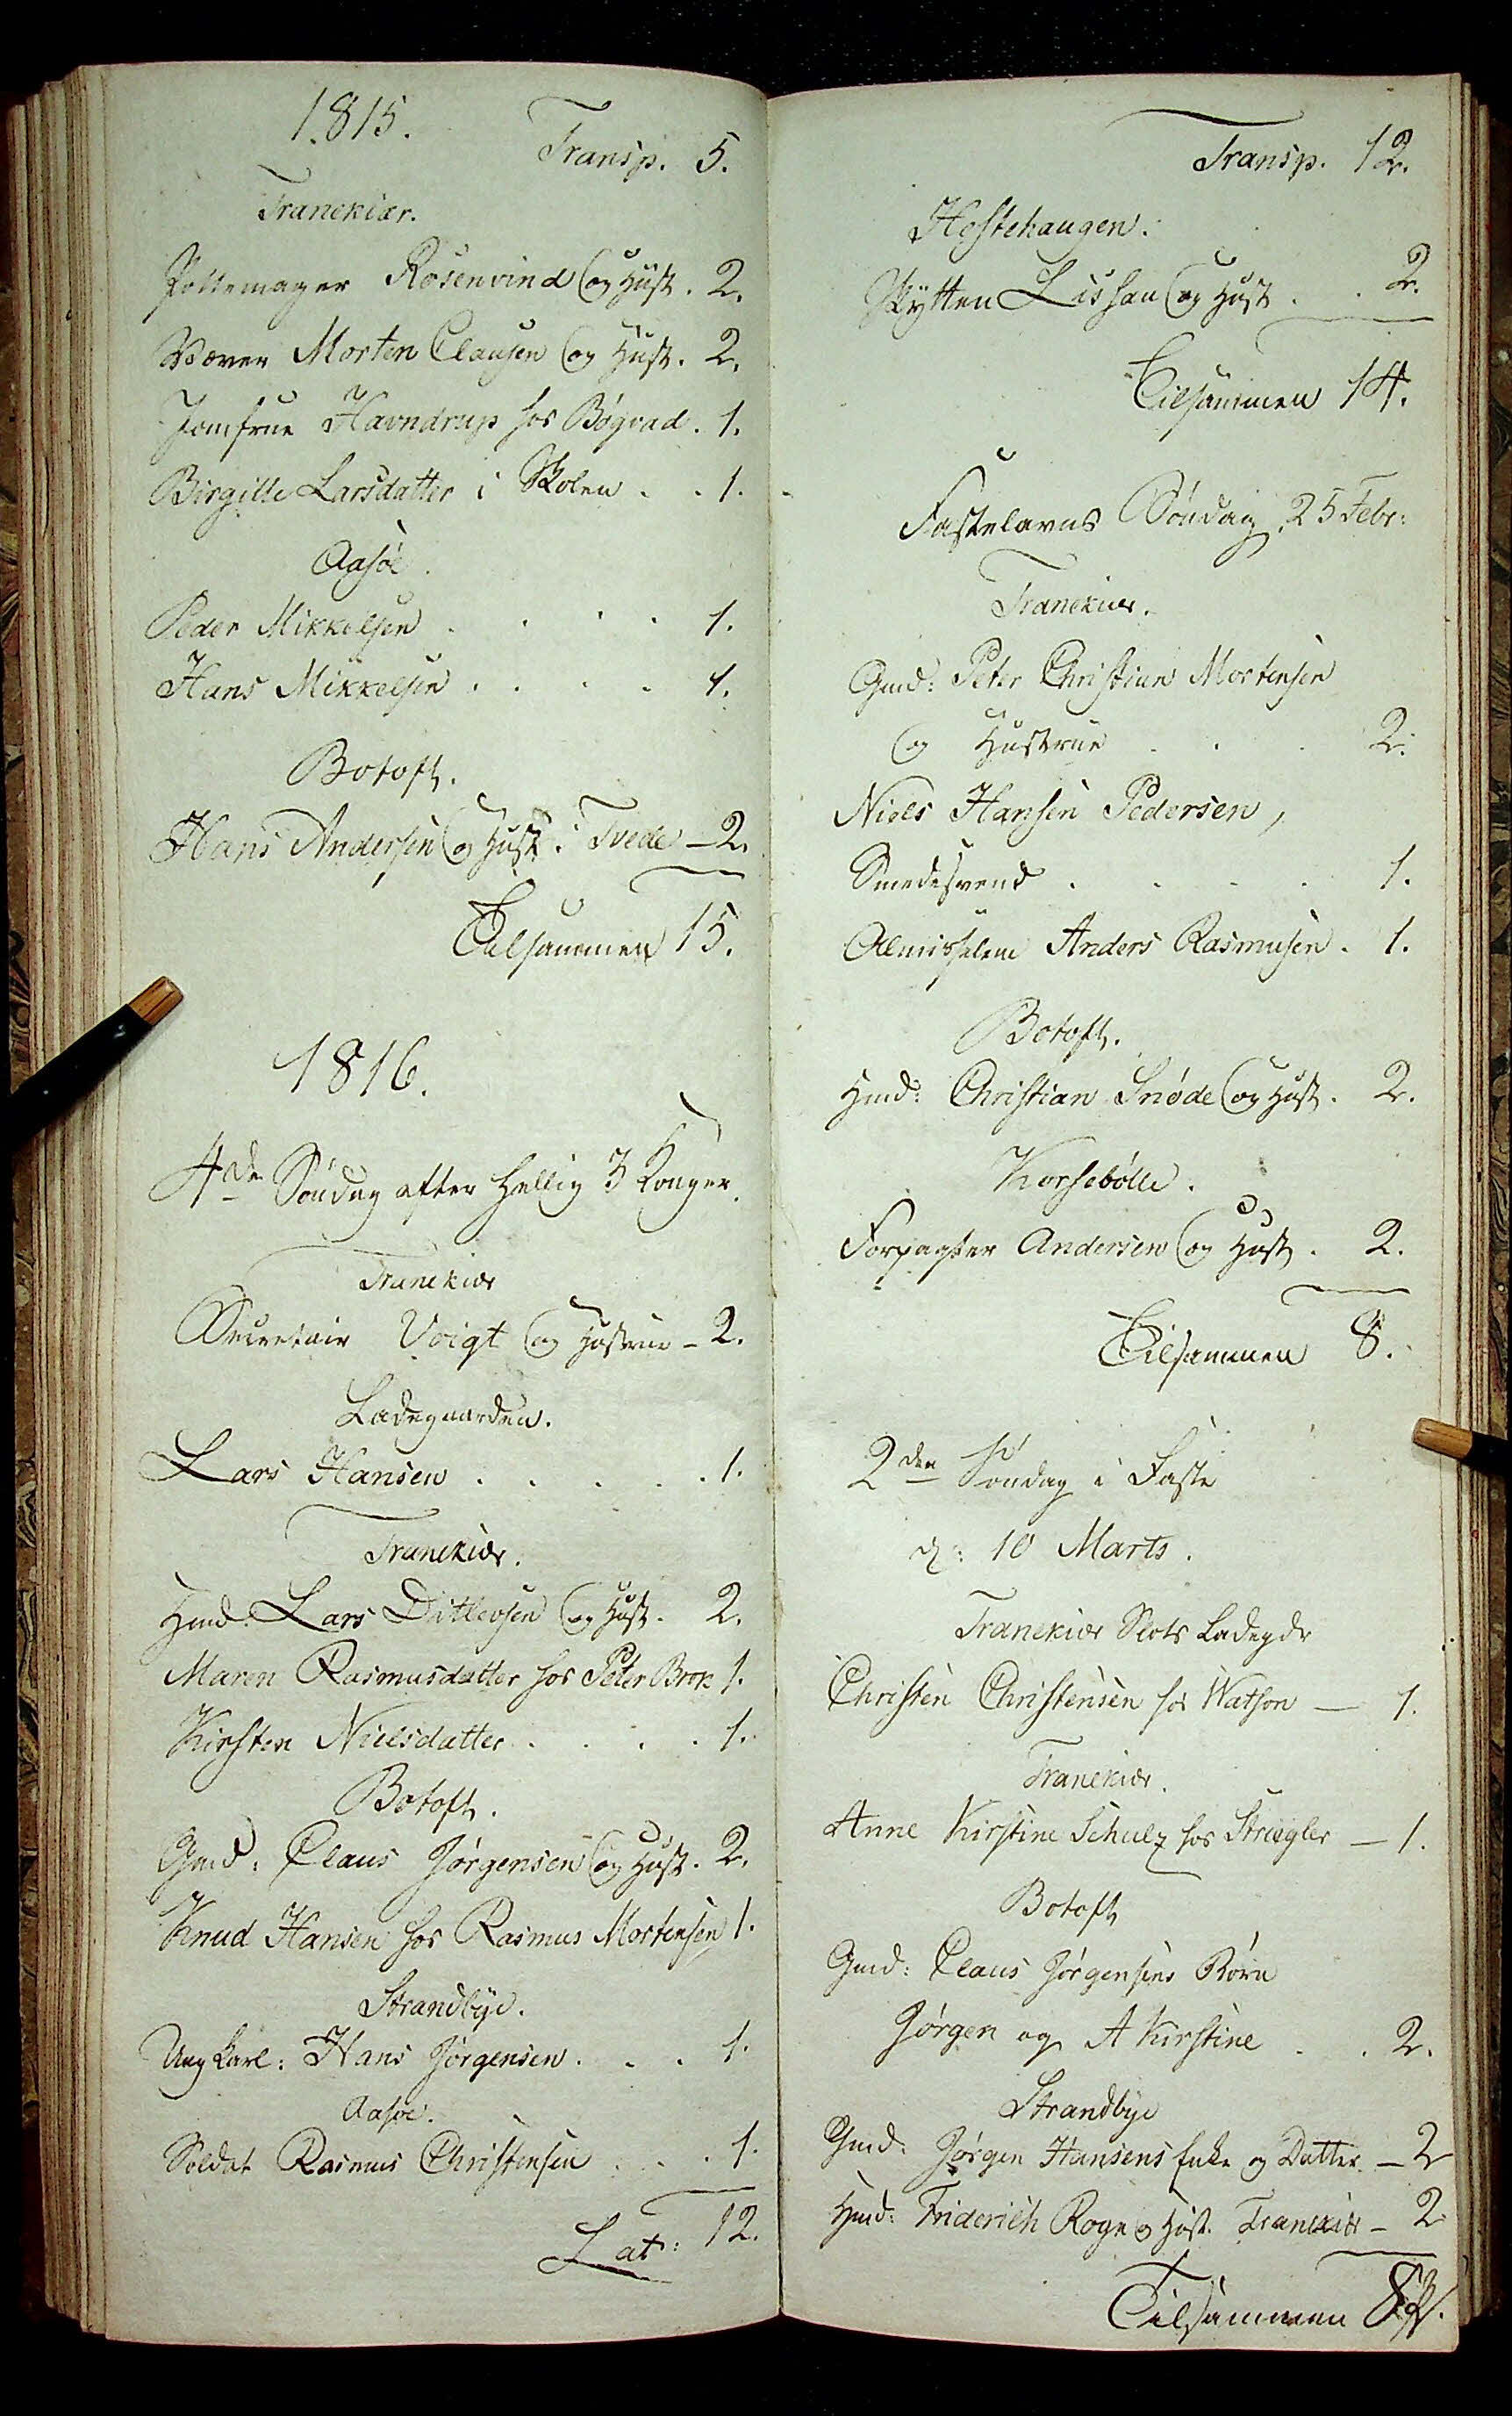1815.
Transp. 5.
Tranekiær.
Pottemager Rosenvind og Hust . 2.
Væver Morten Clausen og Hust . 2.
Jomfrue Havndrup hos Bøgvad . 1.
Birgitte Larsdatter i Skolen . . 1.
Aasøe
Peder Mikkelsen . . . . 1.
Hans Mikkelsen . . . . 1.
Botoft.
Hans Andersen og Hustr i Tvede - 2.
Tilsammen 15.
1816.
4de Søndag efter Hellig 3 Konger
Tranekiær
Secretair Voigt og Hustrue - 2.
Ladegaarden.
Lars Hansen . . . . . 1.
Tranekiær.
Hmd. Lars Ditlevsen og Hust . 2.
Maren Rasmusdatter hos Peter Brok 1.
Kirsten Nielsdatter . . . . 1.
Botoft.
Gmd: Claus Jørgensen og Hust . 2.
Knud Hansen hos Rasmus Mortensen 1.
Strandbye.
Ungkarl: Hans Jørgensen . . . 1.
Aasoe.
Soldat Rasmus Christensen . . . 1
Lat: 12
Transp. 12.
Hestehaugen.
Skytten Lissau og Hust . . 2.
Tilsammen 14.
Fastelavns Søndag 25 Febr:
Tranekiær.
Gmd: Peter Christian Mortensen
og Hustrue . . . 2.
Niels Hansen Pedersen,
Smedesvend . . . . 1.
Almisselem Anders Rasmusen . 1.
Botoft.
Hmd: Christian Snøde og Hust . 2.
Korsebølle.
Forpagter Andersen og Hust . 2.
Tilsammen 8.
2den Søndag i Faste
d: 10 Marts.
Tranekiær Slots Ladegdr
Christen Christensen hos Watson - 1.
Tranekiær
Anne Kirstine Schulz hos Striegler - 1.
Botoft
Gmd: Claus Jørgensens Børn
Jørgen og A Kirstine . . 2.
Strandbye
Gmd: Jørgen Hansens Enke og Datter - 2
Hmd: Friderich Rogn og Hust Tranekiær - 2.
Tilsammen 8 Ps.
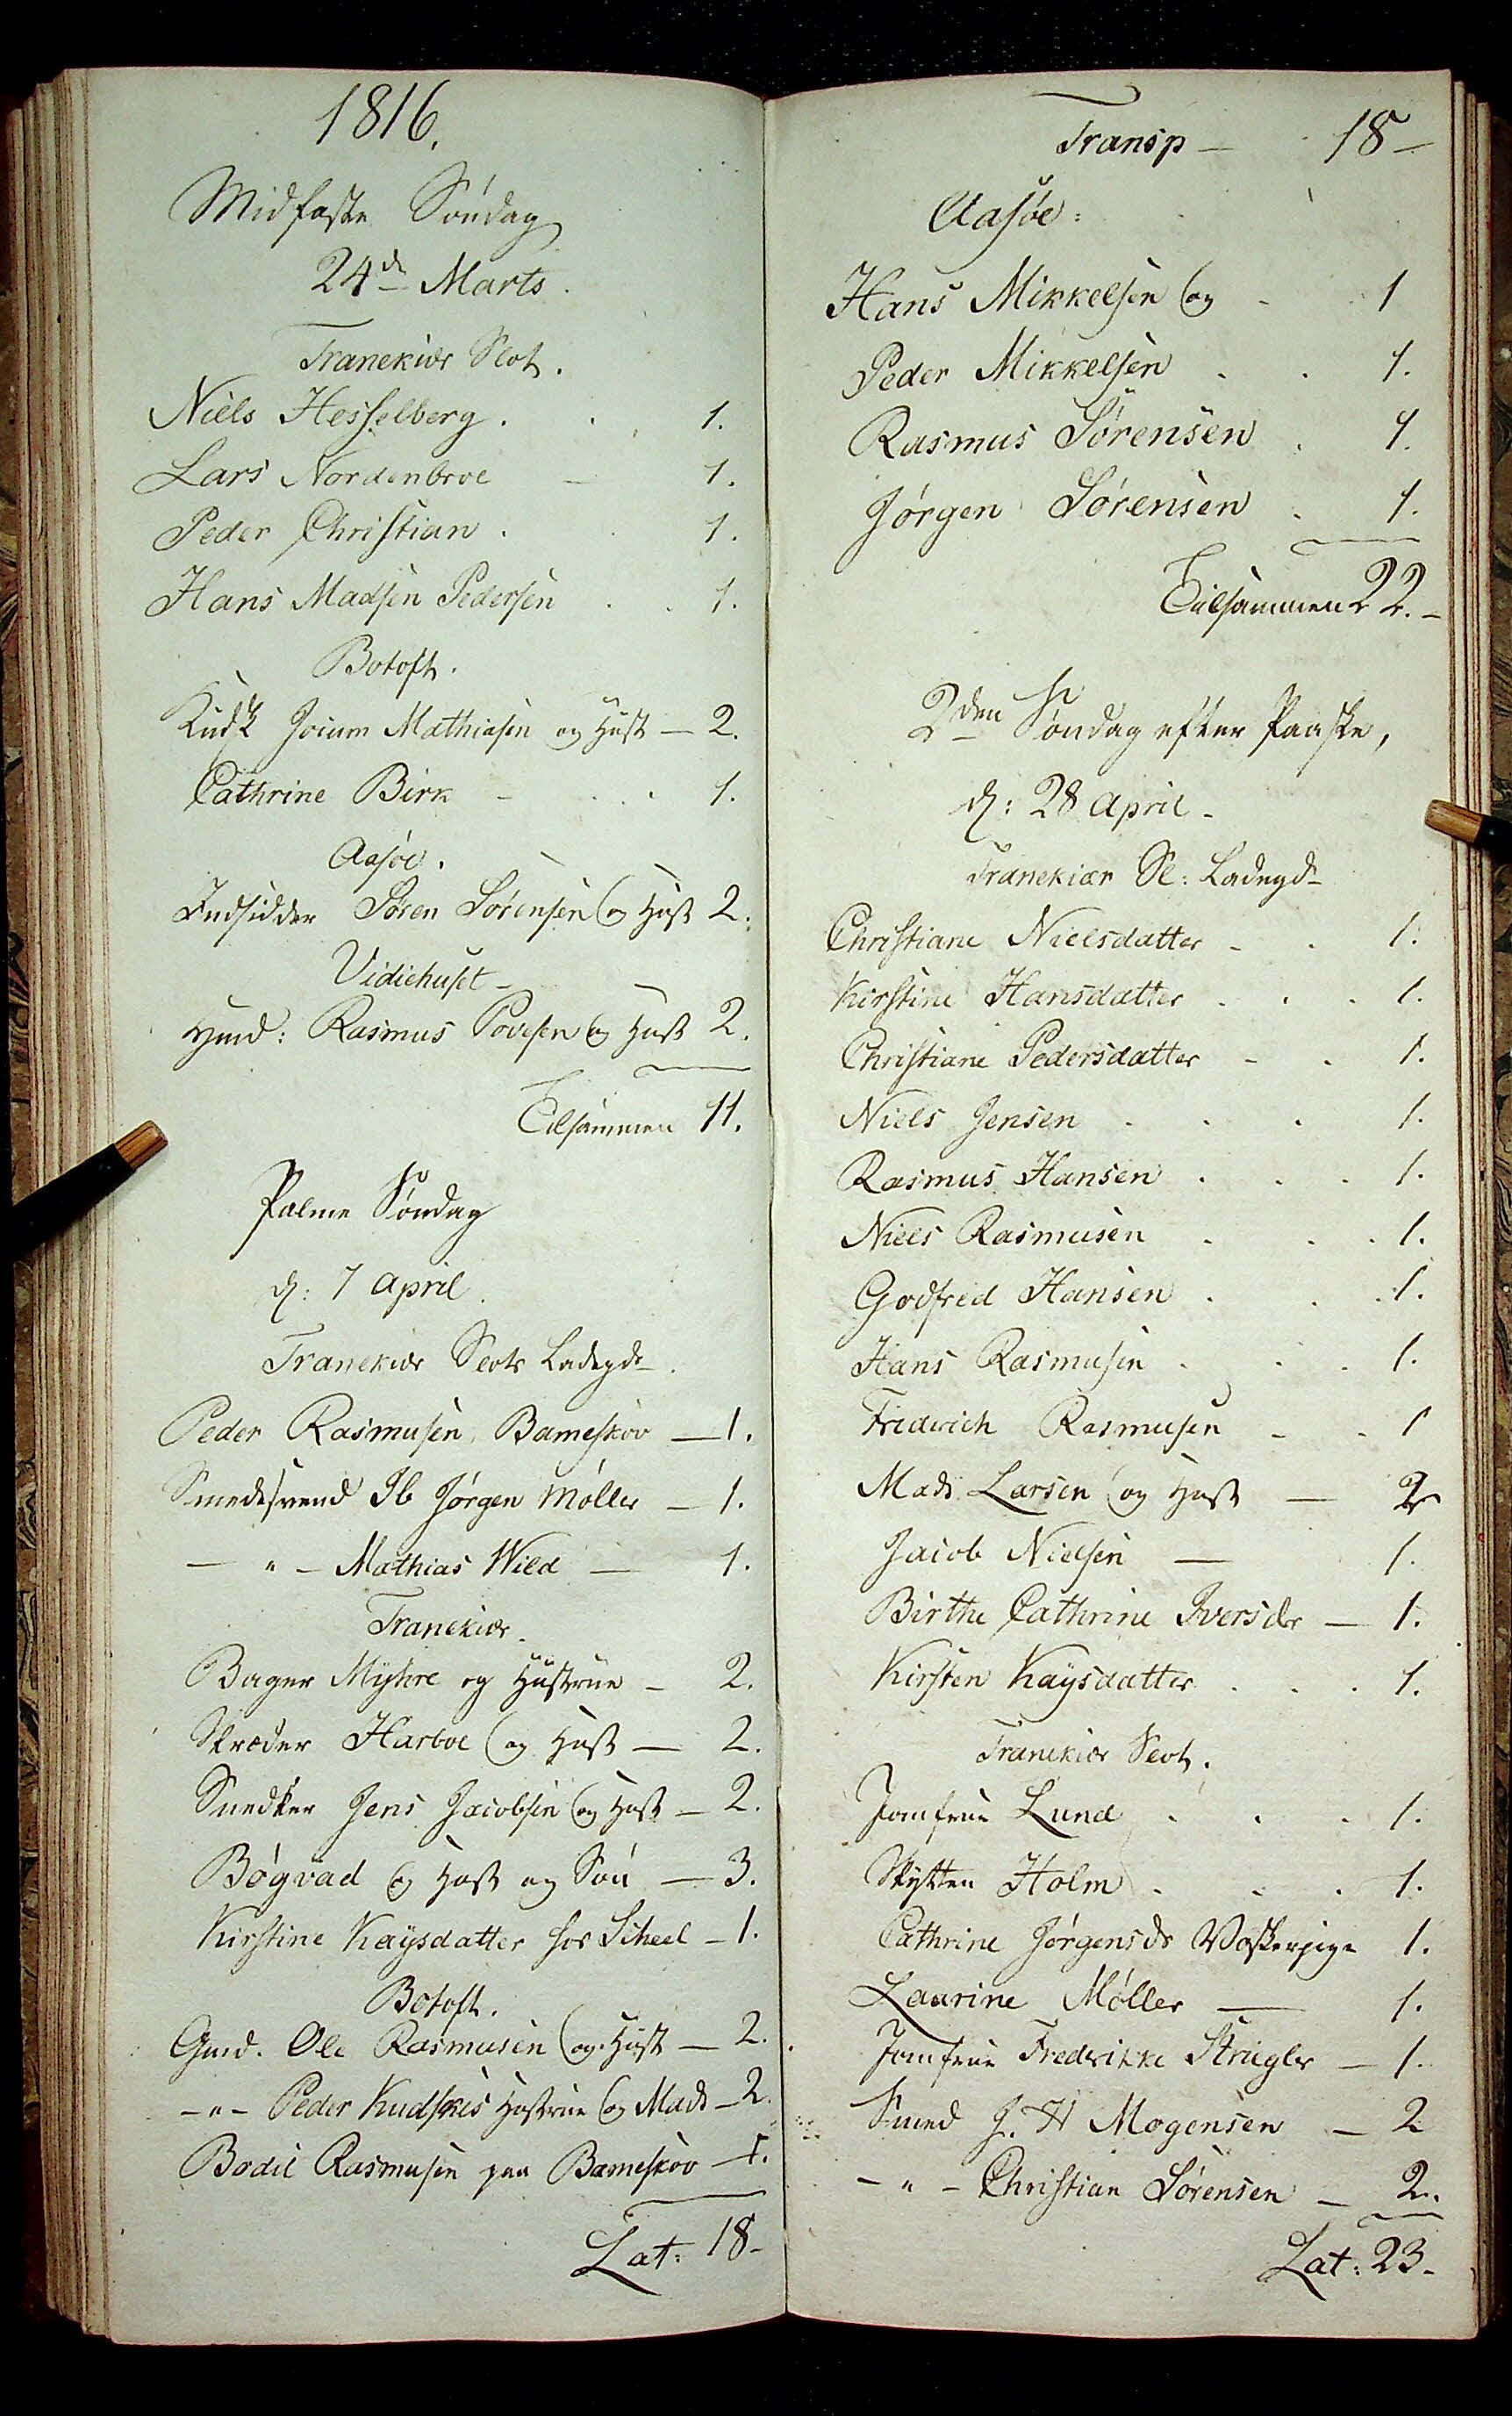1816.
Midfaste Søndag
24de Marts
Tranekiær Slot
Niels Hesselberg . . . 1.
Lars Nordenbroe - 1.
Peder Christian . . 1.
Hans Madsen Pedersen . . 1.
Botoft
Kudsk Jocum Mathiasen og Hust - 2.
Cathrine Birk - . . . 1.
Aasøe.
Indsidder Søren Sørensen og Hustr 2.
Vidiechuset
Hmd: Rasmus Povelsen og Hust 2.
Tilsammen 11.
Palme Søndag.
d: 7 April
Tranekiær Slots Ladegdr
Peder Rasmusen Bameskov - 1.
Smedsvend Ib Jørgen Møller - 1.
-"- Mathias Wild - 1.
Tranekiær
Bager Myhre og Hustrue - 2.
Skræder Harboe og Hust - 2.
Snedker Jens Jacobsen og Hust - 2.
Bøgvad 6 Hust og Søn - 3
Kirstine Kaysdatter hos Scheel - 1.
Botoft.
Gmd. Ole Rasmusen og Hust - 2.
-"- Peder Kudskes Hustrue og Mads - 2
Bodil Rasmusen paa Bameskov - 1.
Lat: 18-
Transp - 18
Aasør:
Hans Mikkelsen og . 1
Peder Mikkelsen . . 1.
Rasmus Sørensen . 1.
Jørgen Sørensen . 1.
Tilsammen 22. -
2den Søndag efter Paaske,
d: 28 April
Tranekiær Sl: Ladegdr
Christiane Nielsdatter . . 1.
Kirstine Hansdatter . . . 1.
Christiane Pedersdatter - . 1.
Niels Jensen . . . 1.
Rasmus Hansen . . . 1.
Niels Rasmusen . . . 1.
Godfred Hansen . . . 1.
Hans Rasmusen . . . 1.
Friderich Rasmusen - . 1
Mads Larsen og Hust - 2
Jacob Nielsen - 1.
Birthe Cathrine Iversdr - 1.
Kirsten Kaysdatter . . 1.
Tranekiær Slot.
Jomfru Lund . . . 1.
Skytten Holm . . . 1.
Cathrine Jørgensdr Vaskerpige 1.
Laurine Møller - 1.
Jomfrue Didrikke Streiegler - 1.
Smed J. H. Mogensen - 2
-"- Christian Sørensen - 2.
Lat:. 23.